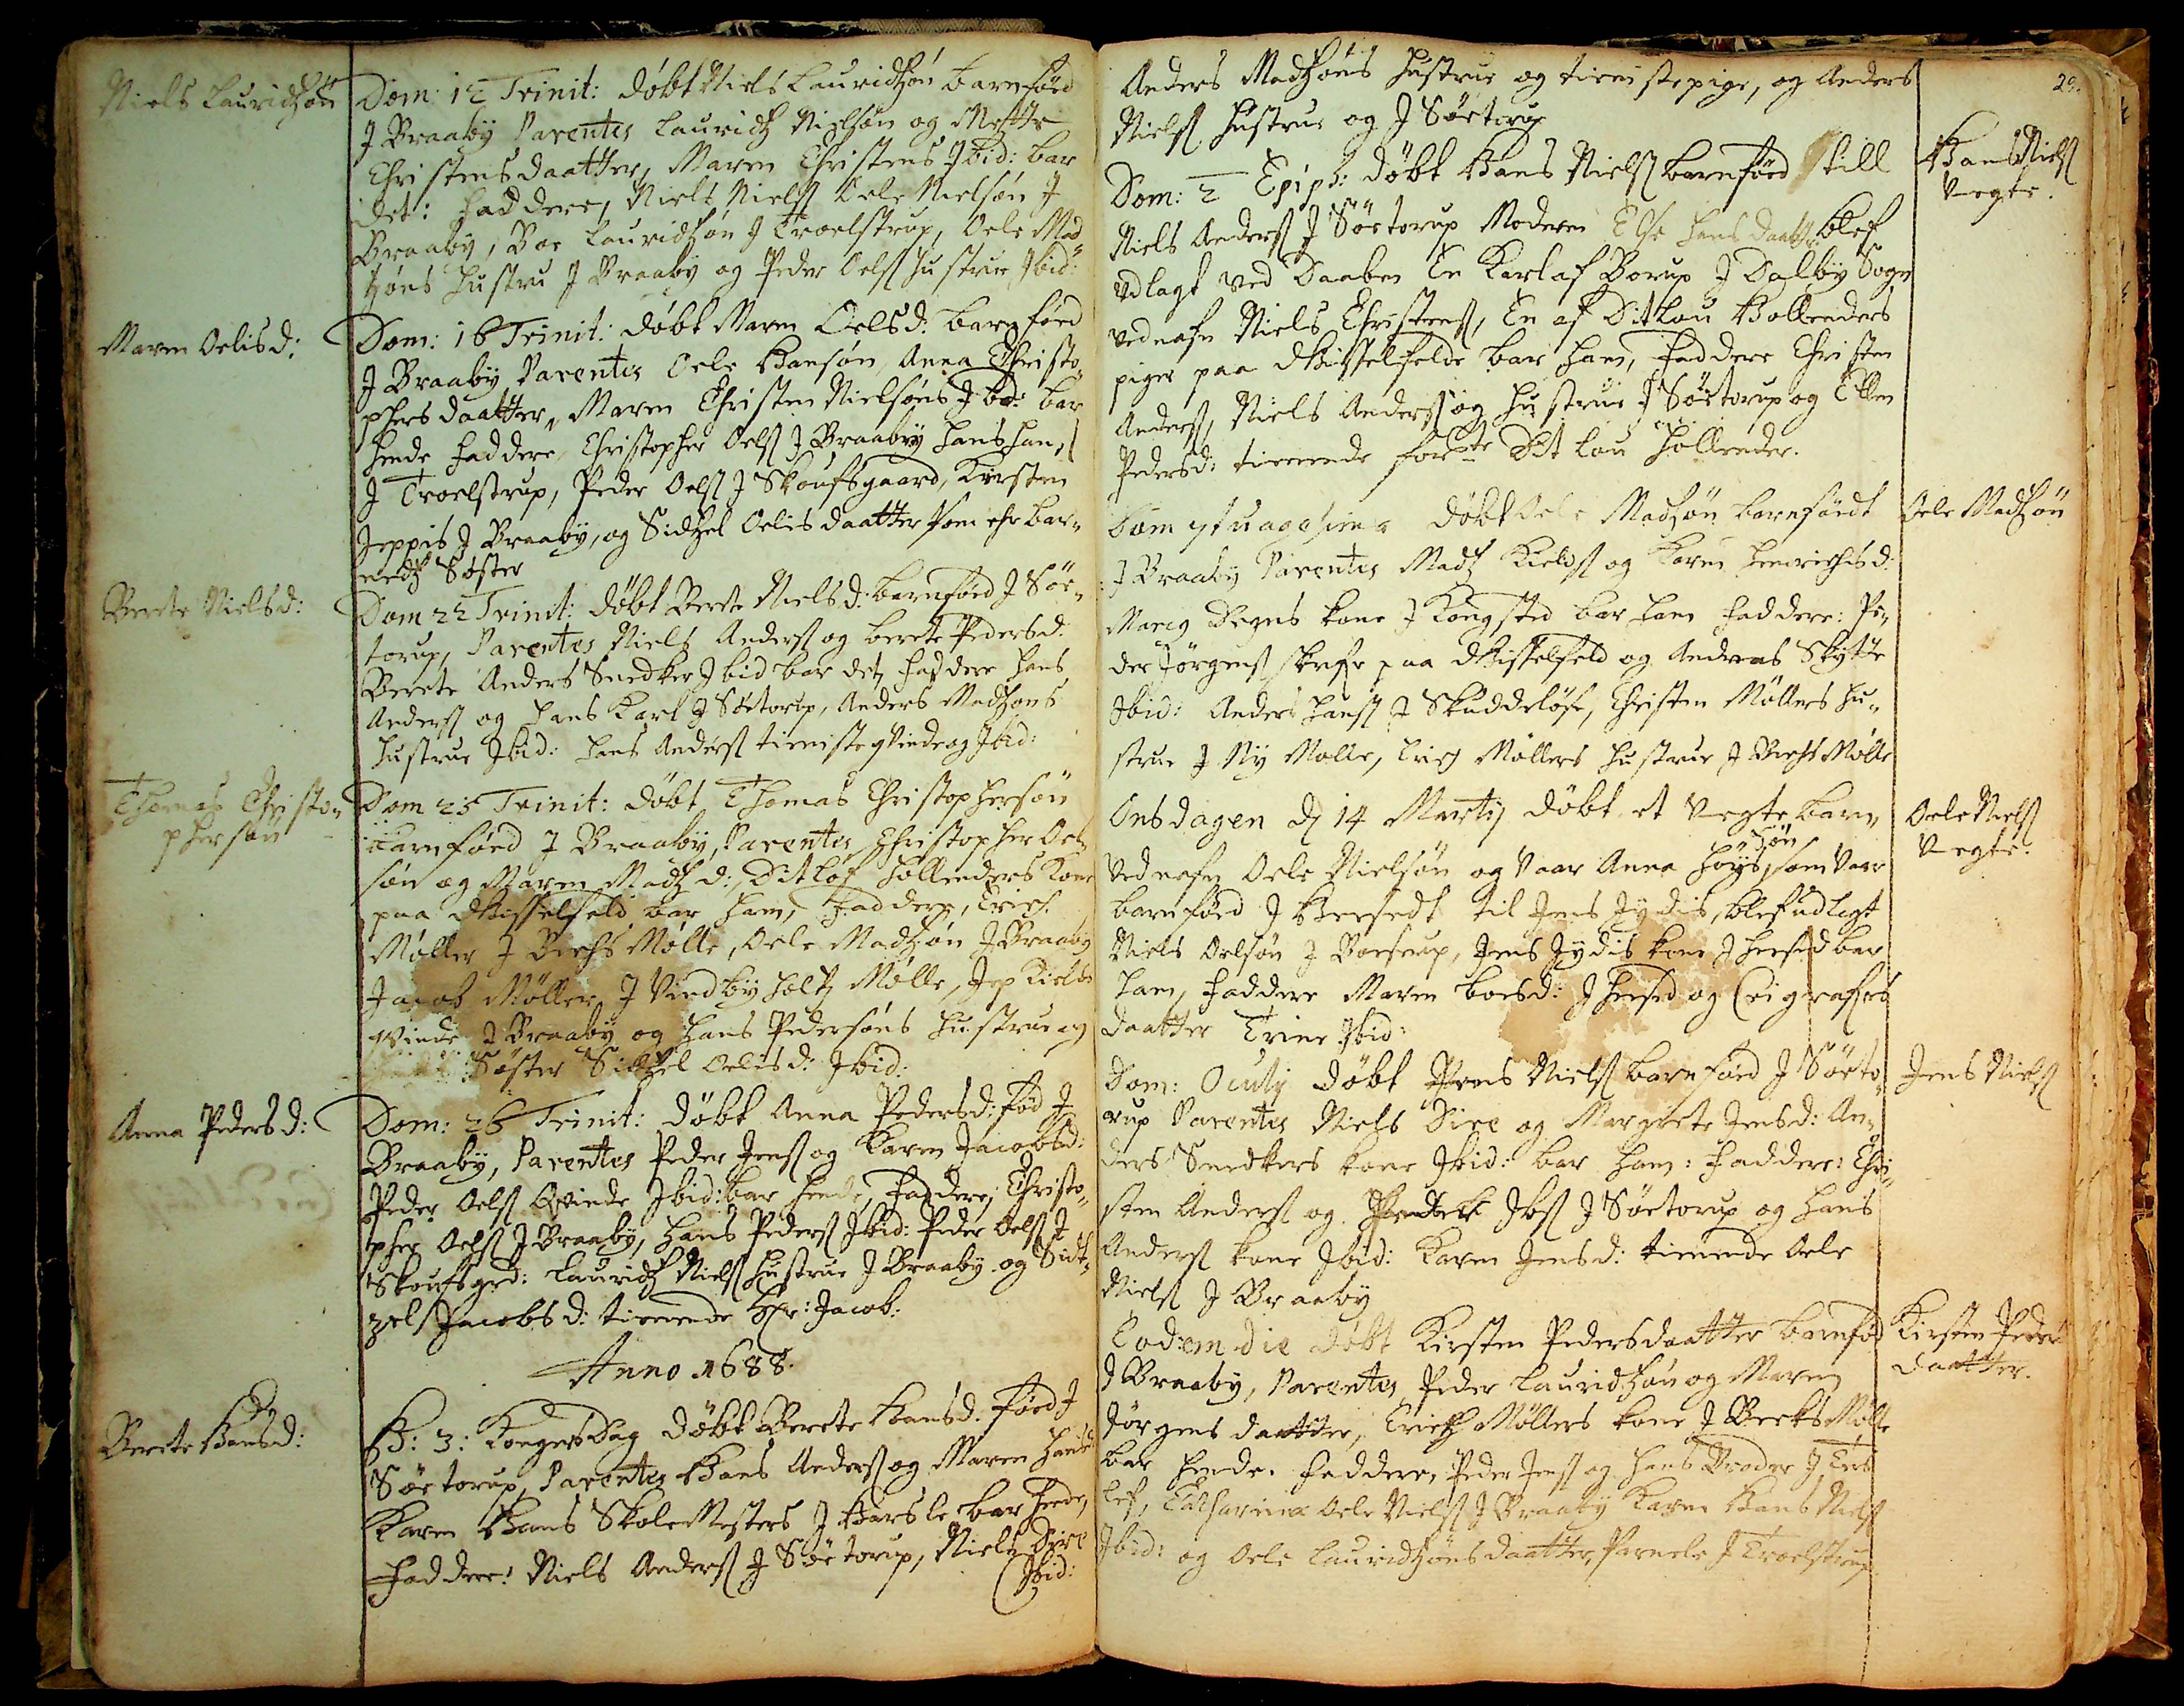Niels Lauridzøn
Dom 12. Trinit: døbt Niels Lauridzøn. barnføed
J Braaby Parentes Laurits Nielsøn og Mette
Christensdaatter, Maren Christens Jbid: bar
det: Faddere, Niels Niels Oele Nielsøn J
Braaby, Boe Lauridsøn J Troelstrup, Oele Mad¬
tzøns Hustru J Braaby og Peder Oels Hustru Jbid.
Maren Oelisd. 
Dom: 16. Trinit: døbt Maren Oelsd. barnføed 
J Braaby Parentes Oele Hansøn, Anne Christo¬
phers daatter, Maren Christen Nielsøns Jid bar
hende. Faddere, Christopher Oels J Braaby, Hans Hans
J Troelstrup, Peder Oels J Skoufsgaard, Kirsten
Jeppis J Braaby, og Sidzel Oelisdaatter som ehr Bar¬
neds søster.
Berete Nielsd. 
Dom. 22. Trinit: døbt Berete Nielsd. Barnføed J Søe¬
torup, Parentes Niels Anders og Berete Pedersd.
Berete Anders Snedker Jbid. bar det, Faddere Hans
Anders og hans Karl J Søetorup, Anders Madsøns
Hustrue Jbid: Hans Anders tienisteqvinde og Jbid:
Thomas Christo¬ 
phersøn
Dom. 25. Trinit: døbt Thomas Christophersøn 
barnføed J Braaby, Parentes Christopher Oele
søn og Maren Madsd:, Ditløf Hollenders Kone
paa Gisselfeld bar ham, Faddere, Erich
Møller J Bechs Mølle, Oele Madsøn J Braaby
Jacob Møller J Vindbyholtz Mølle, Jep Kields
qvinde J Braaby og Hans Pedersøns Hustrue og
Søster Sidsel Oelisd: Jbid:
Anna Pedersd. 
Dom. 26. Trinit: døbt Anna Pedersd. fød J 
Braaby, Parentes: Peder Jens og Karen Jacobsd.
Peder Oels Qvinde Jbid. bar hende, Faddere, Christo¬
pher Oels J Braaby, Hans Peders Jbid. Peder Oels J
Skoufsgrd: Lauritz Niels hustrue J Braaby og Sid¬
zel Jacobsd. tienende Hxr: Jacob:
Anno 1688
Berete Hansd:
H. 3 Kongers Dag døbt Berete Hansd. føed J 
Søetorup Parentes Hans Anders og Maren Hansd.
Karen Hans Skolemesters i Harsle bar hende. 
Faddere; Niels Anders J Søetorup, Niels Dire
Jbid:
Anders Madsøns hustrue og tienestepige, og Anders 
Niels Hustrue og J Søetorup
29.
Dom: 2 Epiph: døbt Hans Niels barnføed till
Niels Anders J Søetorup Moderen Else Hans daatter. Blef
udlagt ved Daaben En Karl af Borup J Dalby Sogn
ved nafn Niels Christens, En af Ditlou Hollenders
piger paa Gisselfelde bar ham, Faddere Christen
Anders, Niels Anders og Hustrue J Søetorup og Ellen
Pedersd. tienende forte Ditlou Hollender.
Hans Niels
U-egte
Dom 7tuagesima døbt Oele Madsøn barnføedt J
Braaby Parentes Mads Kielsds og Karen Henrichsd:
Mary Degns kone J Kongsted bar ham faddere: Pe¬
der Jørgens skrifr. paa Gisselfeld og Anders Skytte
Jbid: Anders Hans J Skuddeløse, Christen Møllers hu¬
strue J Ny Mølle, Erich Møllers Hustrue, J Bechs Mølle.
Oele Madsøn
Onsdagen d. 14 Marty døbt et uegte barn  
Søn
ved nafn Oele Nielsøn og vaar Anna Høys, som vaar
barnføed J Heesedt til Jens Jydis, blef udlagt
Niels Oelsøn J Boeserup, Jens Jydis kone J Heesed bar
ham, Faddere Maren Boesd: J Heesed og Cai grafrs
datter Trine Jbid:
Oele Niels
U-egte.
Dom: Oculy døbt Jens Niels barnføed J Søeto¬
rup Parentes Niels Dire og Margrete Jensd: An¬
ders Snedkers Kone Jbid: bar ham: Faddere: Chri¬
sten Anders og Peder Jbsøn J Søetorup og Hans
Anders kone Jbid: Karen Jensd: tienende Oele
Niels J Braaby. 
Jens Niels 
Eodem die døbt Kirsten Pedersdaatter barnfød 
J Braaby, Parentes Peder Lauridsøn og Maren
Jørgens daatter, Erich Møllers kone J Beeks Mølle 
bar hende. Faddere, Peder Jens og hans Broder J Ters
lef, Catharina Oele Niels J Braaby Karen Hans Niels
Jbid: og Oele Lauridsøns daatter, Parnels## J Troelstrup.
Kirsten Peders
datter.
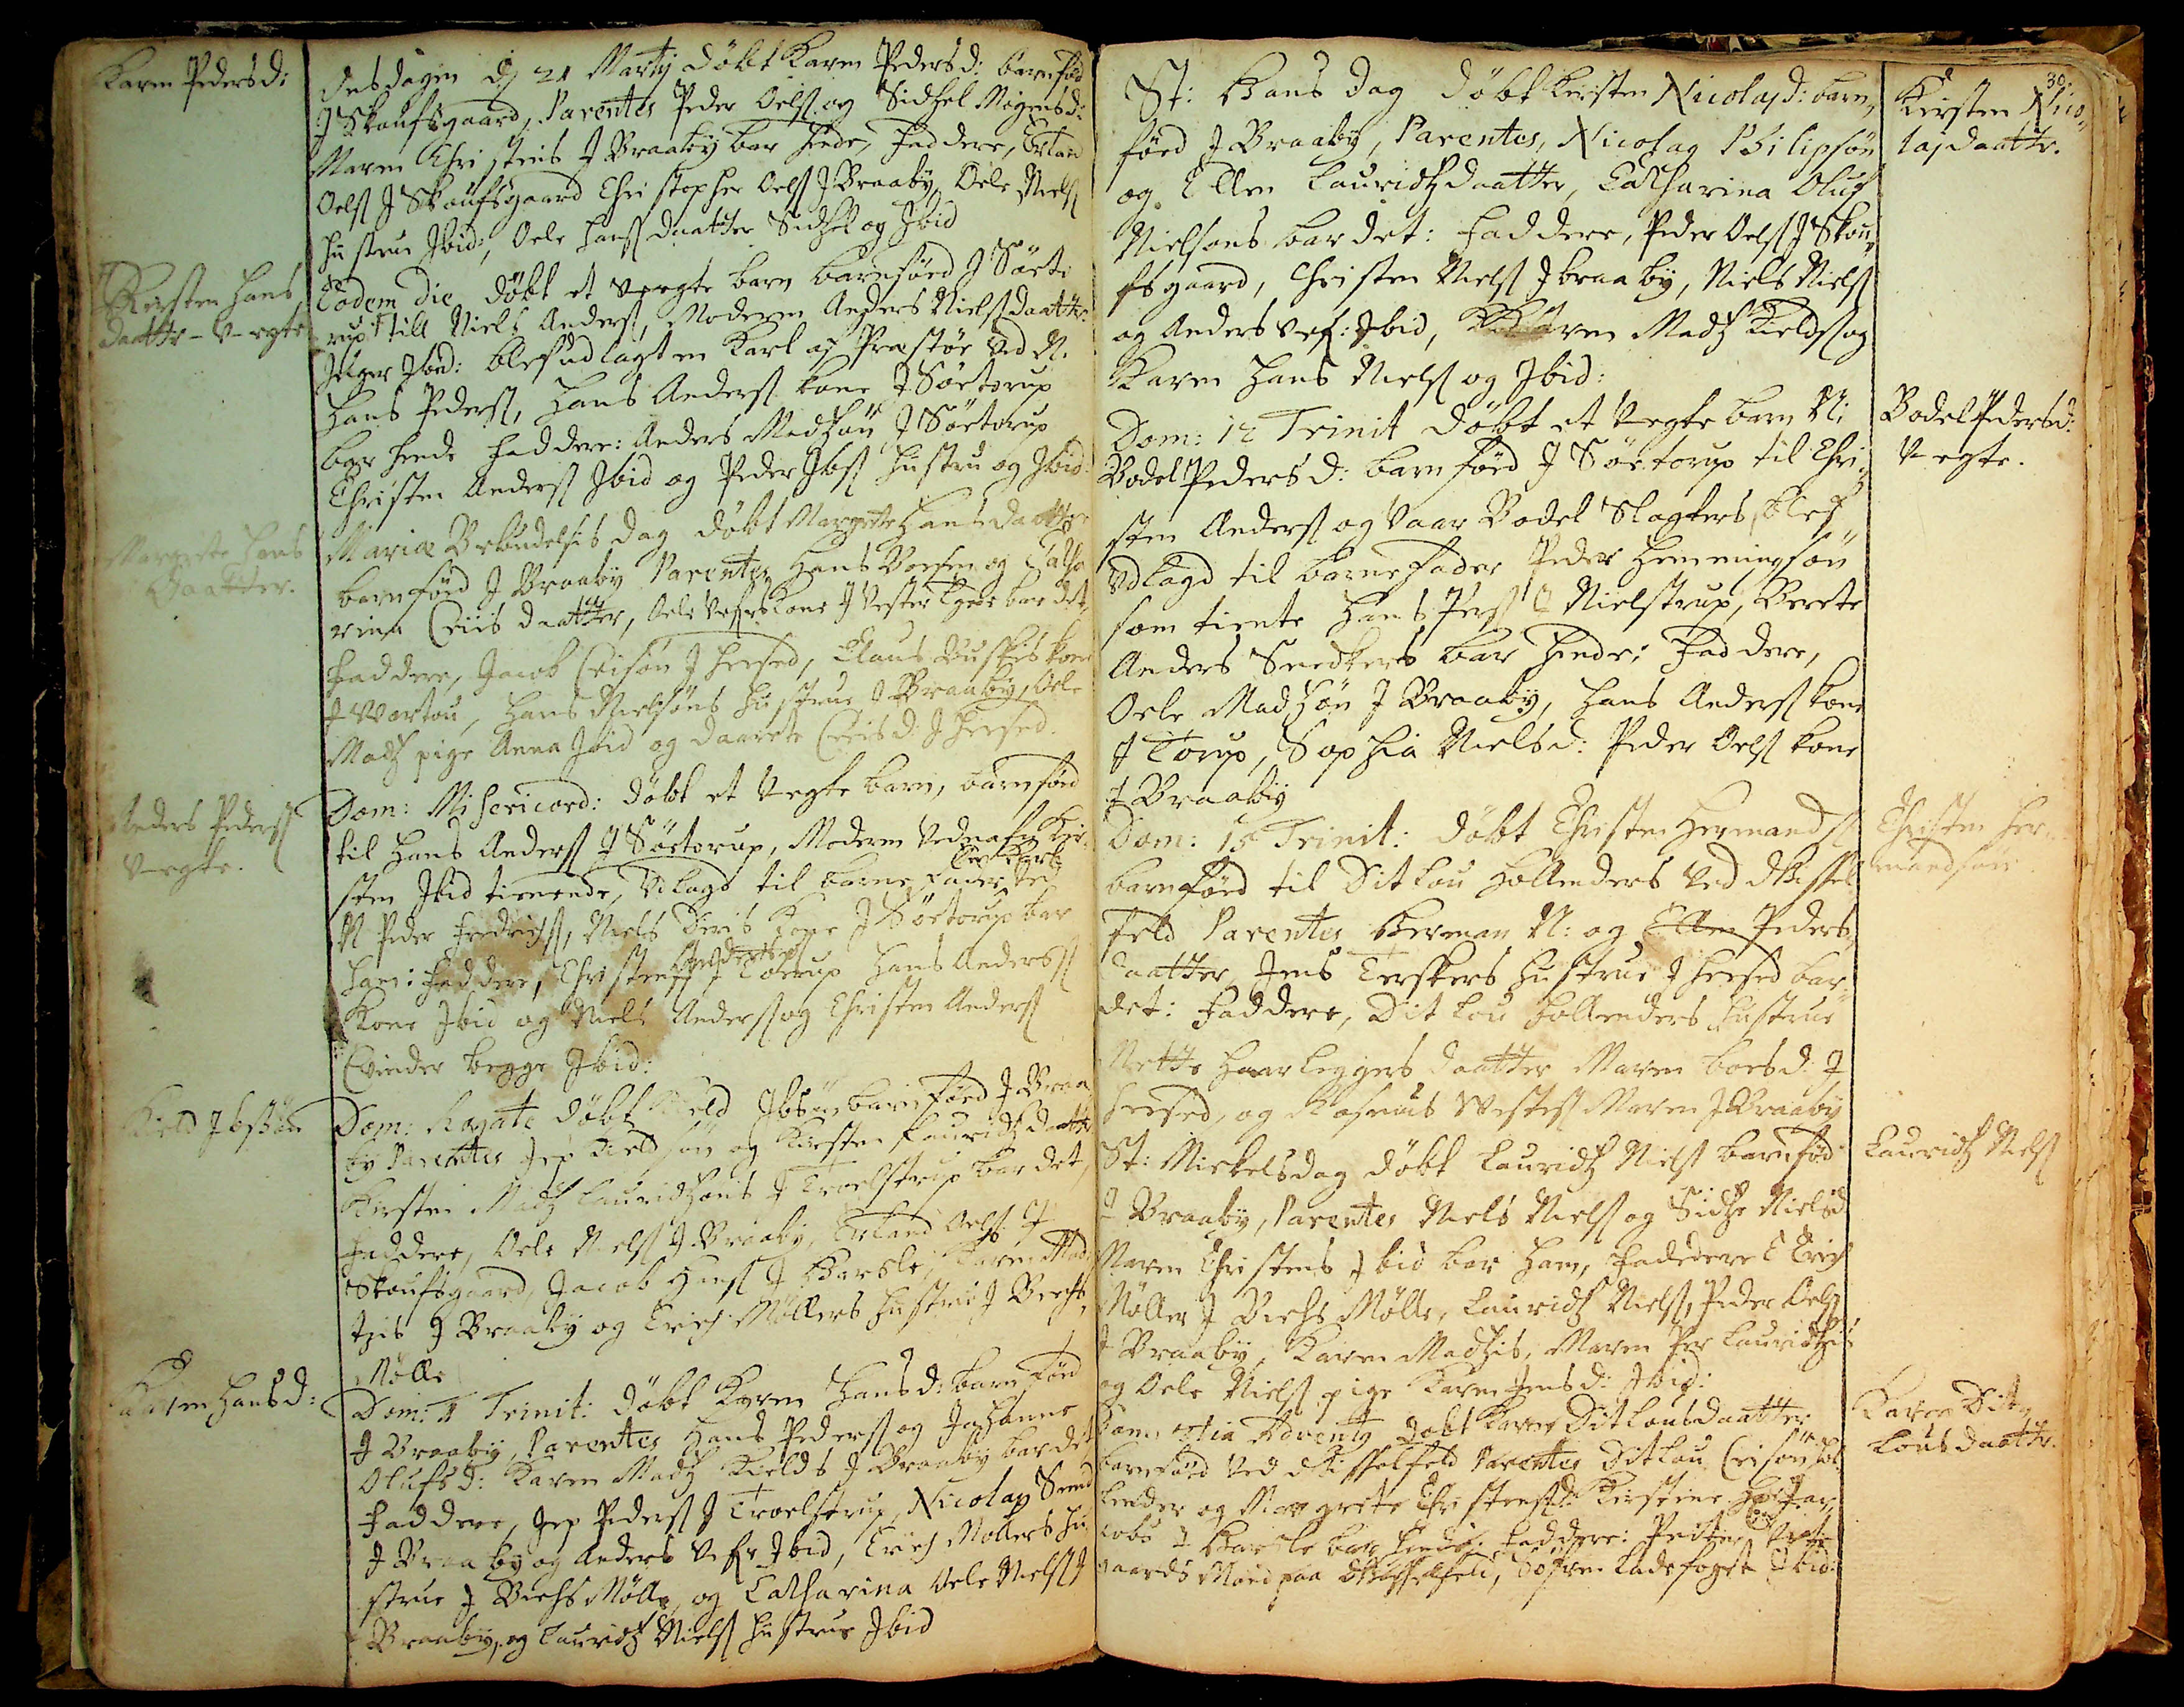Karen Pedersd. 
Onsdagen d. 21 Marty døbt Karen Pedersd: barnføed
J Skoufsgaard, Parentes Peder Oels og Sidsel Mogensd:
Maren Christens J Braaby bar hende, Faddere, Erland
Oels J Skoufsgaard, Christopher Oels J Braaby, Oele Niels
Hustrue Jbid. Oele Hansøns daatter Sidsel og Jbid.
Kirsten Hans
datter - U-egte
Eodem Die døbt et Ueegte barn barnføed J Søeto
rup till Niels Anders, Moderen Anders Niels daatter.
Jnger Jbid: blef udlagt en Karl af Præstøe ved N.
Hans Peders, Hans Anders Kone J Søtorup
bar hende Faddere: Anders Madsøn J Søetorup
Christen Anders Jbid og Peder Jbs Hustru og Jbid.
Margrete Hans 
Daatter 
Maria Bebudelsis dag døbt Margrete Hansdaatter,
barnføed J Braaby Parentes, Hans Boesen og Cath
erine Ceiis daatter, Oele Veffers Kone J Vester Egede bar det.
Faddere, Jacob Ceisøn J Heesed, Klaus Buskis Kone
i Vartou, Hans Nielsøns hustrue J Braaby, Oele
Mads pige Anna Jbid og Daarete Ceeisd. J Heesed.
Anders Peders
U-egte.
Dom: Misericord: døbt et Uegte barn, barnføed 
til Hans Anders J Søetorup, Moderen Ved Nafn Kir¬
en Karl
sten Jbid. tienende, Udlagt til barnefader ved
N. Peder Fredericks, Niels Deris Kone J Søetorup bar
Andersøn
ham: Faddere Christen J Toerup, Hans Anders
Kone Jbid. og Niels Anders og Christen Anders
Qvinder begge Jbid:
Kield Jbsøn 
Dom: Rogate døbt Kield Jbsøn barnføed J Braa
by Parentes Jep Kieldsøn og Kirsten Lauridtz daatter
Kirsten Mads Lauridsøns J Troelstrup bar det.
Faddere, Oele Niels J Braaby, Erland Oels J
Skoufsgaard, Jacob Hans J Harsle, Karen Mad¬
tzis J Braaby og Erich Møllers Hustru J Bechs
Mølle. 
Karen Hansd: 
Dom: 1 Trinit: døbt Karen Hansd: barn føed
J Braaby, Parentes, Hans Peders og Johanne
Olufsd: Karen Mads Kields J Braaby bar det
Faddere, Jep Peders J Troelstrup, Nicolaj Smid
J Braaby og Anders Vefer Jbid., Erich Møllers Hu
strue J Bechs Mølle og Catharina Oele Niels J
 Braaby og Lauridz Niels Hustrue Jbid.
Sct. Hans Dag døbt Kirsten Nicolajd: barn¬
føed J Braaby, Parentes, Nicolaj Philipsøn
og Ellen Lauridsdaatter, Katharina Oluf
Nielsens bar det: Faddere, Peder Oels J Skou¬
fsgaard, Christen Niels J Braaby, Niels Niels
og Anders Vef: Jbid, Karen## Mads Kieldsøn, og
Karen Hans Niels og Jbid:
30.
Kirsten Nico¬
lajdaatter
Dom. 12 Trinit døbt et Uegte barn N:
Bodel Pedersd: Barn føed J Søetorup til Chri¬
sten Anders og vaar Bodel Slagters, blef
udlagt til barnefader Peder Hemmingsøn
som tiente Hans Jens J Nielstrup, Berete
Anders Snedhers bar hende; Faddere,
Oele Madsøn J Braaby, Hans Anders Kone
J Torup, Sophia Nielsd. Peder Oels Kone
J Braaby.
Bodel Pedersd: 
U-egte
Dom: 15 Trinit: døbt Christen Hermands
barnføed til Ditlou Hollenders ved Gissel
feld Parentes Herman N. og Ellen Peders
daatter, Jens Terskers Hustrue J Hersed bar
det; Faddere, Ditlou Hollenders Hustrue
Mette Haar Leggers## daatter Maren Boesd. J
Hersed, og Rasmus Vestes Maren J Braaby.
Christen Her¬ 
mandsøn 
St: Miekelsdag døbt Laurids Niels barnfød
J Braaby, Parentes Niels Niels og Sidse Nielsd.
Maren Christens Jbid bar ham. Faddere: E. Erich
Møller J Bechs Mølle, Lauridtz Niels, Peder Oels
J Braaby, Karen Madsis, Maren Per Lauridsis
og Oele Niels, pige Karen Jensd: Jbid:
Lauridtz Niels
Dom. 3tia Adventy døbt Karen Ditlous daatter
barnføed ved Gisselfeld Parentes Ditlou Ceisøn Hol¬
lender og Margrete Christensd. Kirstine Hxr. Jar¬
cobs J Harsle bar hende. Faddere: Peiter Urte
gaards Mand paa Gisselfeld, Søfren Ladefoget Jbid:
Karen Dit
lous daatter